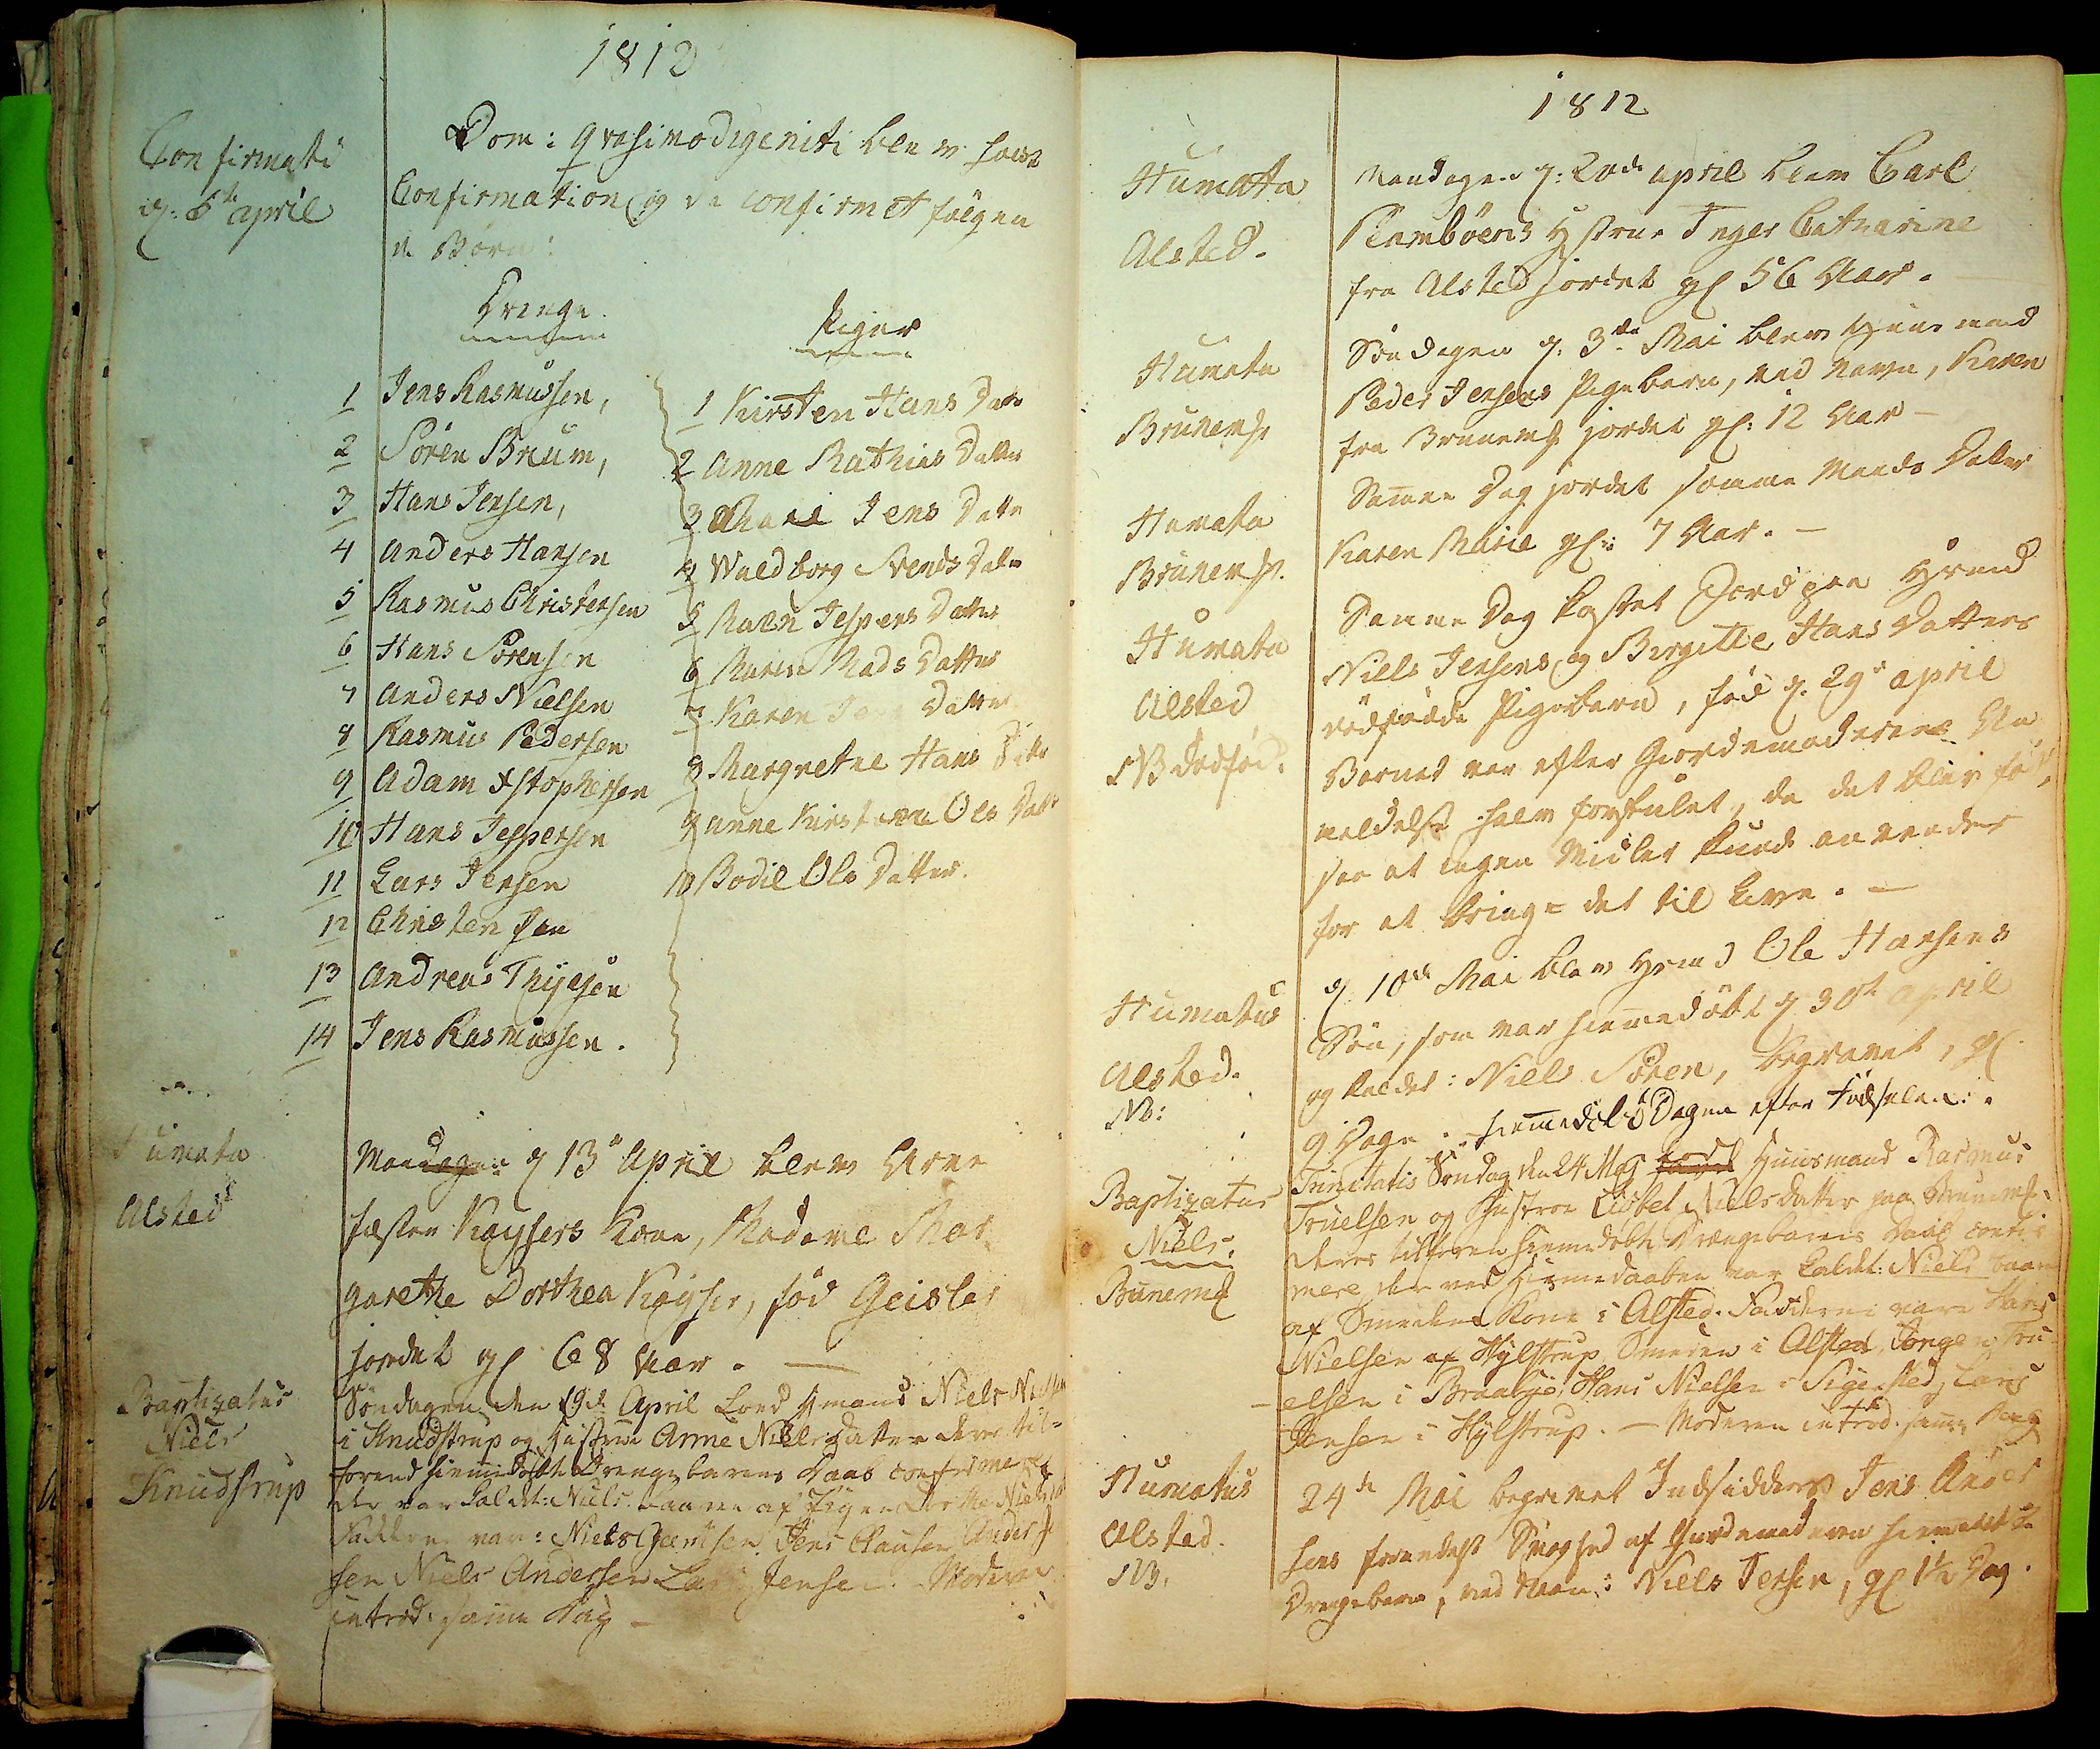Confirmati
d: 5te April.
Humata
Alsted
Baptizatua
Niels
Knmudstrup
1812
Dom: Qvasimodogeniti blev v holdt
Confirmation og da confirmet følgen
de Børn
Drenge
1 Jens Rasmussen,
2 Søren Brium,
3 Hans Jensen,
4 Anders Hansen
5 Rasmus Christensen
6 Hans Sørensen
7 Anders Nielsen
8 Rasmus Pedersen
9 Adam Xstophersen
10 Hans Jespersen
11 Lars Jensen
12 Christen Jen
13 Andreas Thyesen
14 Jens Rasmussen
Piger
1 Kirsten Hans Datter
2 Anne Mathias Datter
3 Marie Jens Datter
4 Waldborg Svends Datter
5 Maren Jespers Datter
6 Maren Madsdatter
7 Karen Jens Datter
8 Margrethe Hans Datter
9 Anne Kirst## Ols Datter
10 Bodil Ols Datter
Mandagen d 13de April blev Arve
fæster Kaysers Kone, Madame Mar
garethe Dorthea Kayser, fød Geisler
jordet gl 68 Aar.
Søndagen den 19de April loed Hmand Niels Nielsen
i Knudstrup og Hustrue Anne Niels Datter deres til¬
forend hiemmedøbt Drengebarns Daab confirmere
der var kaldet: Niels. baaren af Pigen Dorthe Niel##
Fadderne vare: Niels Svensen, Jens Clausen Anders J
sen, Niels Andersen Lars Jensen. Moderen
introd: samme Dag
Humata
Allsted.
Humata
Brunem##
Humata
Brunem##.
Humata
Alsted
NB dødfød.
Humatus
Alsted.
NB:
Baptizatus
Niels
Brunem##
Humatus
Alsted.
NB.
1812
Mandagen d: 20de April blev Carl
Peambøens Hustrue Inger Catharine
fra Alsted jordet gl 56 Aar.
Søndagen d: 3de Mai blev Huusmand
Peder Jensens Pigebarn, ved Navn, Karen
fra Brunem## jordet gl: 12 Aar
Samme Dag jordet samme Mands Datter
Karen Marie gl: 7 Aar.
Samme Dag fastet Jord paa Hsmd
Niels Jensens og Birgitte Hans Datter
dødfødde Pigebarn, fød d: 29de April
Barnet var efter Giordemoderens An
meldelse halv forstrulet, da det blev født,
saa at ingen Midler kunde anvendes
for at bringe det til Live.
d: 10de Mai blev Hsmd Ole Hansens
Søn, som var hiemedøbt d 30te April
og kaldet: Niels Søren, begravet, gl
9 Dage Hiemmedøbt Dagen efter Fødselen.
lod
Trinitatis Søndag d 24 Maj havde Huusmand Rasmus
Truelsen og Hustroe Lisbet Niels Datter paa Brunem##
deres tilforn hiemedøbte Drængebarns Daab confir
mere der ved Hiemedaaben var kaldet: Niels baaren
af Smedens Kone i Alsted. Fadderne vare Hans
Nielsen af Hylstrup Smeden i Alsted, Jørgen Tru
elsen i Braaby, Hans Nielsen i Sigersed, Lars
Jensen i Hylstrup. Moderen introd: samme Dag
24de Mai begravet Indsidder Jens Ander¬
sens formedelst Svaghed af Giordemoderen hiemmedøbte
Drengebarn, ved Navn: Niels Jensen, gl 1½ Dag
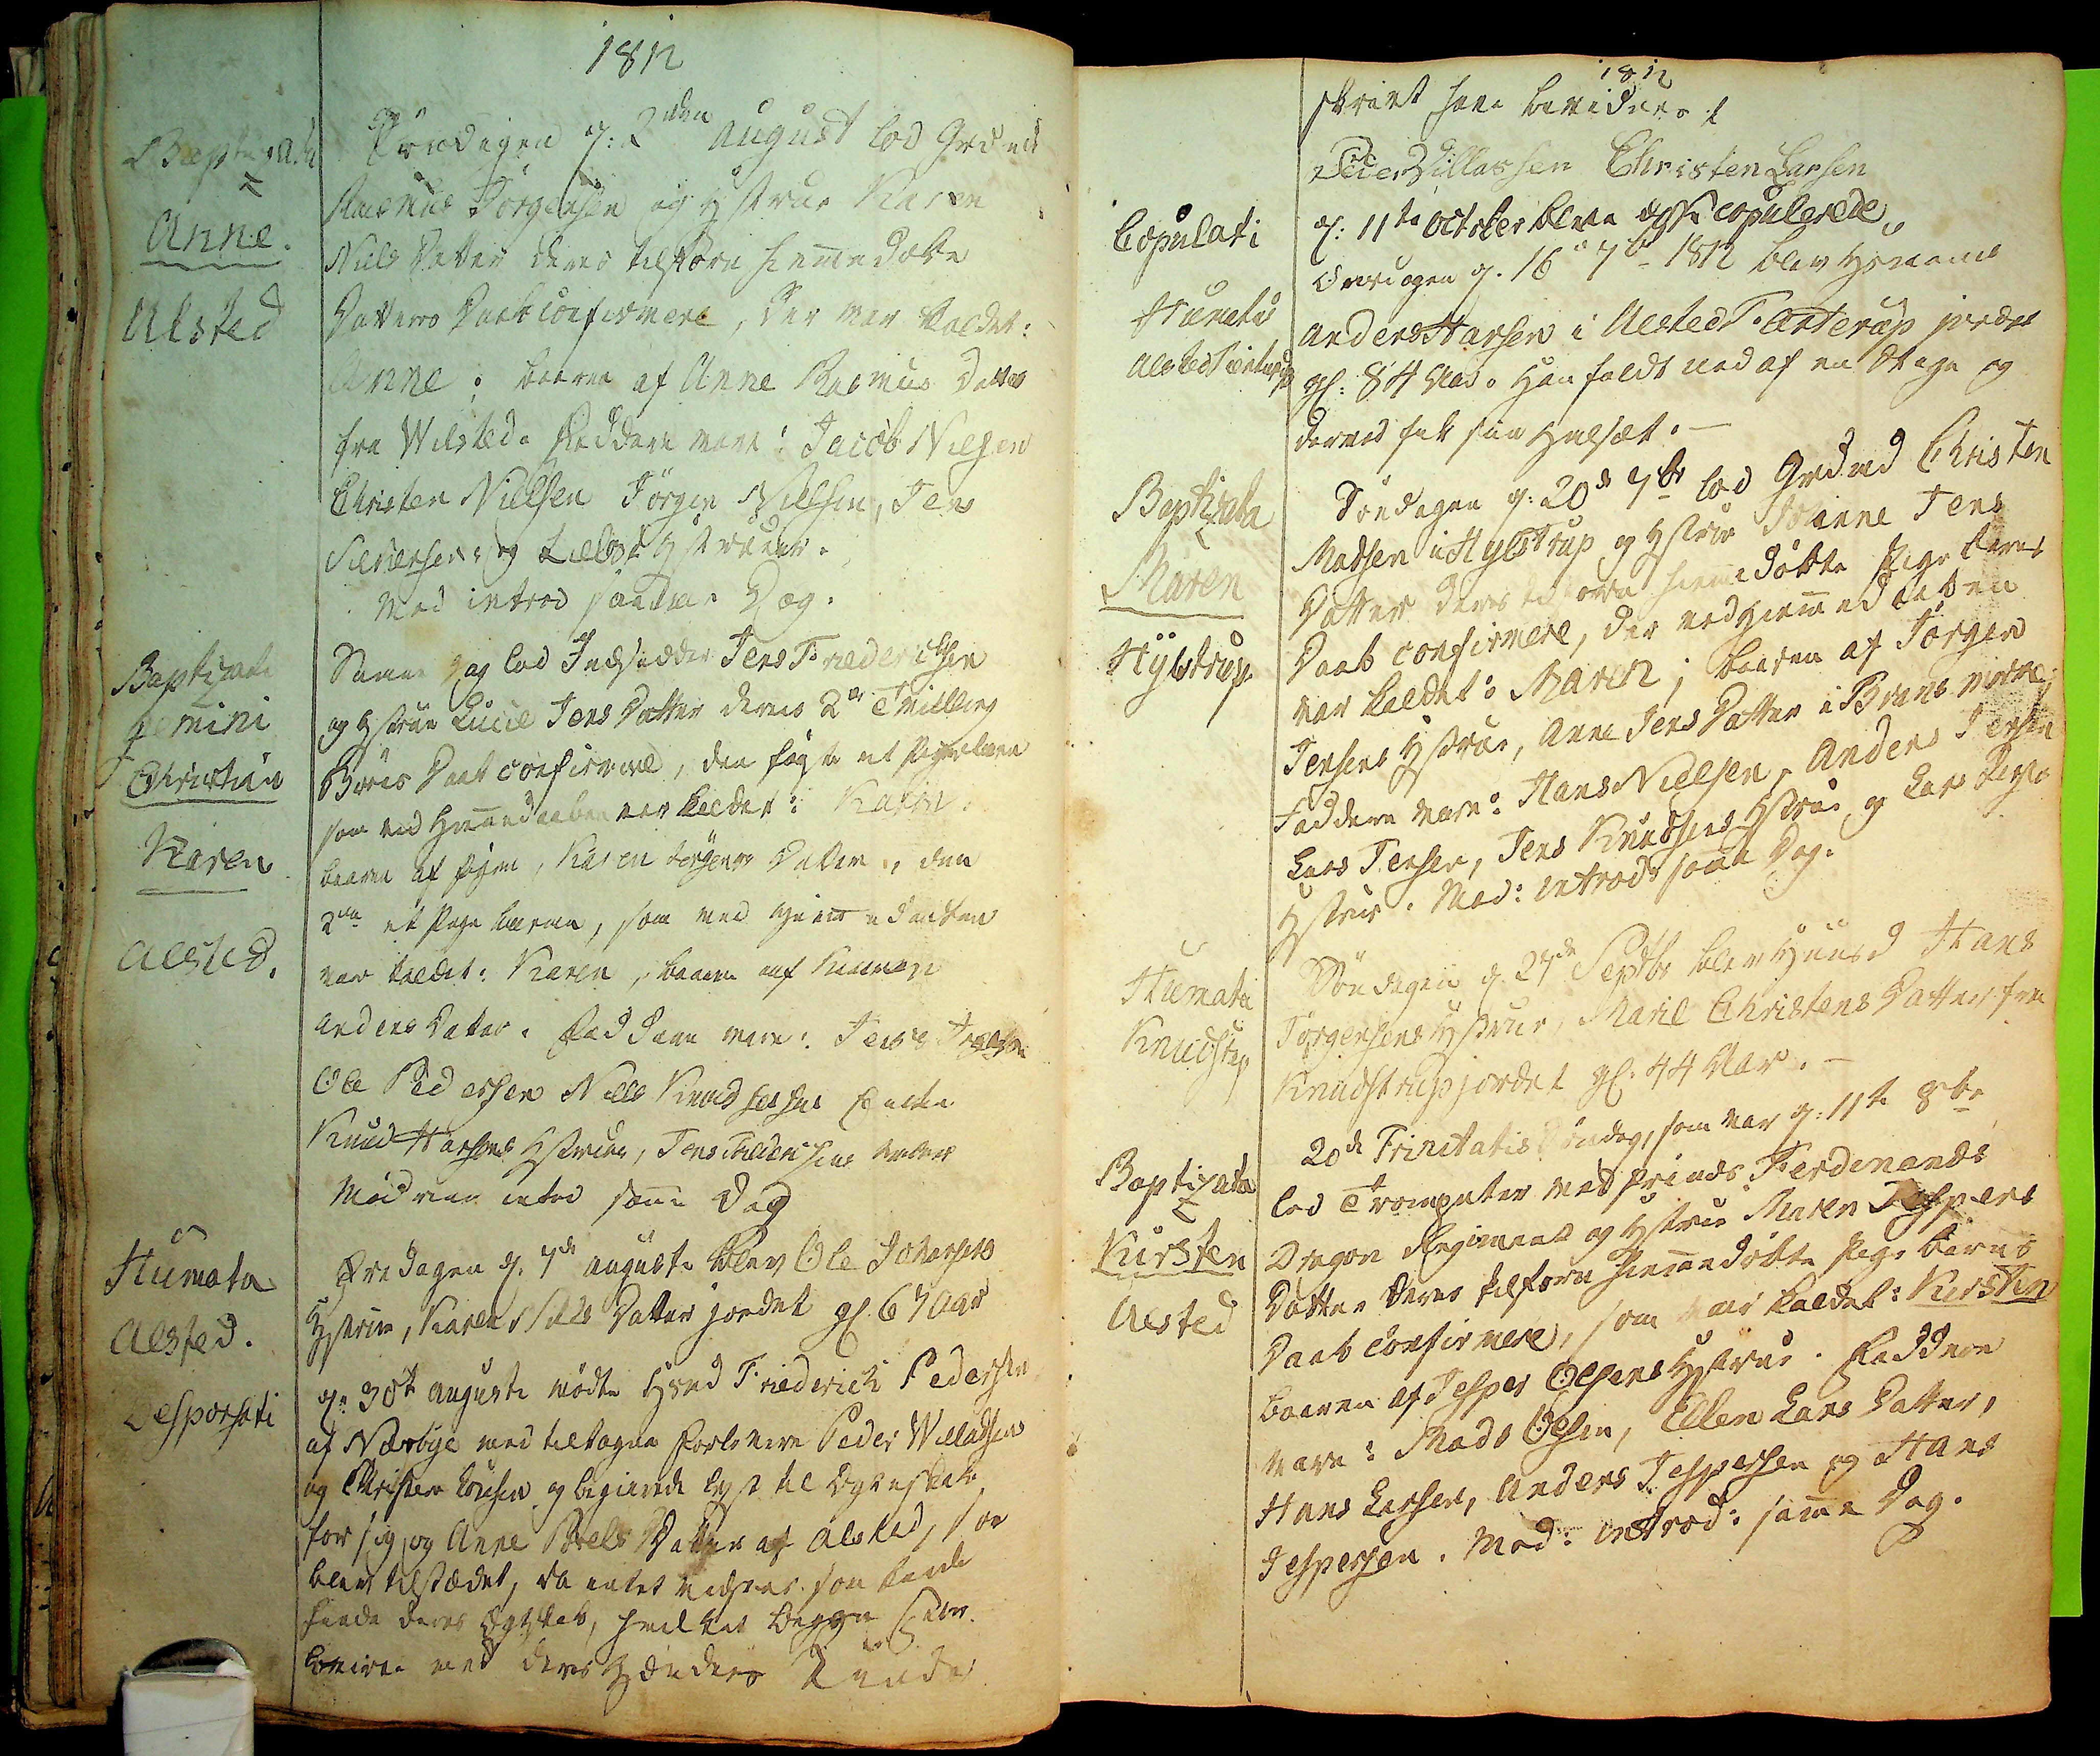Baptizata
Anne
Alsted
Baptizati
gemini
Christian
Karen
Alsted
Humata
Alsted.
Desponsati
1812
Søndagen d: 2den August lod Grmd
Rasmus Jørgensen og Hustrue Karen
Niels Datter haves tilforn hiemmedøbte
Datters Daab confirmere, der var kaldet
Anne; baarern af Anne Rasmus Datter
fra Wilsted. Faddere vare: Jacob Nielsen
Christen Nielsen Jørgen Nielsen, Jens
Silkensen og Liebsts Hstrue
Mod introd samme Dag.
Samme Dag lod Indsidder Jens Frederichsen
og Hustrue Lucie Jens Datter deres 2de Tvilling
Børns Daab confirmere, den første et Pigebarn
som ved Hiemmedaaben var kaldet: Karen
baaren af Pigen Karen Jørgens Datter, den
2den et Pige barn, som ved hiemmedaaben
var kaldt: Karen, baaren af Karen
Anders Datter. Fadderne vare: Jens J##
Ole Pedersen Niels Knudsessns Enke
Knud Hansens Hustrue, Jens Frederichsens Moder
Moderen introd samme Dag
Fredagen d. 7de Augusti blev Ole Johansens
Hustrue, Karen Niels Datter jordet gl 67 Aar
d. 30te Augusti mødte Hsmd Friderich Pedersen
af Næsbye med tiltagne Forlovere Peder Willadsen
og Christen Larsen og begierede lyst til Ægteskab
for sig, og Anne Povelss Datter af Alsted, som
blev tilstædet, da intet vidstes som kunde
hindre deres Ægtskab, hvilket begge For
lovere med deres Hænders Under
Copulati
Humatus
Alsted Flinterup
Baptizata
Maren
Hylstrup
Humata
Knudstrup
Baptizata
Kirsten
Alsted
1812
skrivt have bevidenet
Peder Villassen Christen Larsen
d: 11te October blev disse copulerede
Onsdagen d: 16de 7br 1812 blev Hsmand
Anders Hansen i Alsted Flinterup jordet
gl: 84 Aar. Han foldt ned af en Stige og
derved fik sin Hvesæt.
Søndagen d: 20de 7br lod Grdmd Christen
Madsen ai Hylstrup og Hstrue Johanne Jens
Datter deres til forn hiemmedøbte Pigebarns
Daab confirmere, der ved Hiemmedaaben
var kaldet: Maren; Baaren af Jørgen
Jensens Hstrue Snne Jens Datter i Brunemarken
Fadere vare: Hans Nielsen, Anders Jensen
Lars Jensen, Jens Knudsens Hstrue og Lars Jensens
Hstrue. Mod: introd samme Dag.
Søndagen d 27de Septbr blev Huusd Hans
Jørgensens Hustrue Marie Christens Datter fra
Knudstrup jordet gl: 44 Aar.
20de Trinitatis Søndag, som var d: 11te 8br
lod Trompeter, ved Prinds Ferdenands
Dragon Regiment og Hstrue Maren Jespers
Datter deres tilforn hiemmedøbte Pigebarns
Daab confirmere, som var kaldet: Kirsten
baaren af Jesper Olsens Hstrue. Faddere
vare: Mads Olsen, Ellen Lars Datter,
Hans Larsen Anders Jespersen og Hans
Jespersen. Mod: introd: samme Dag.